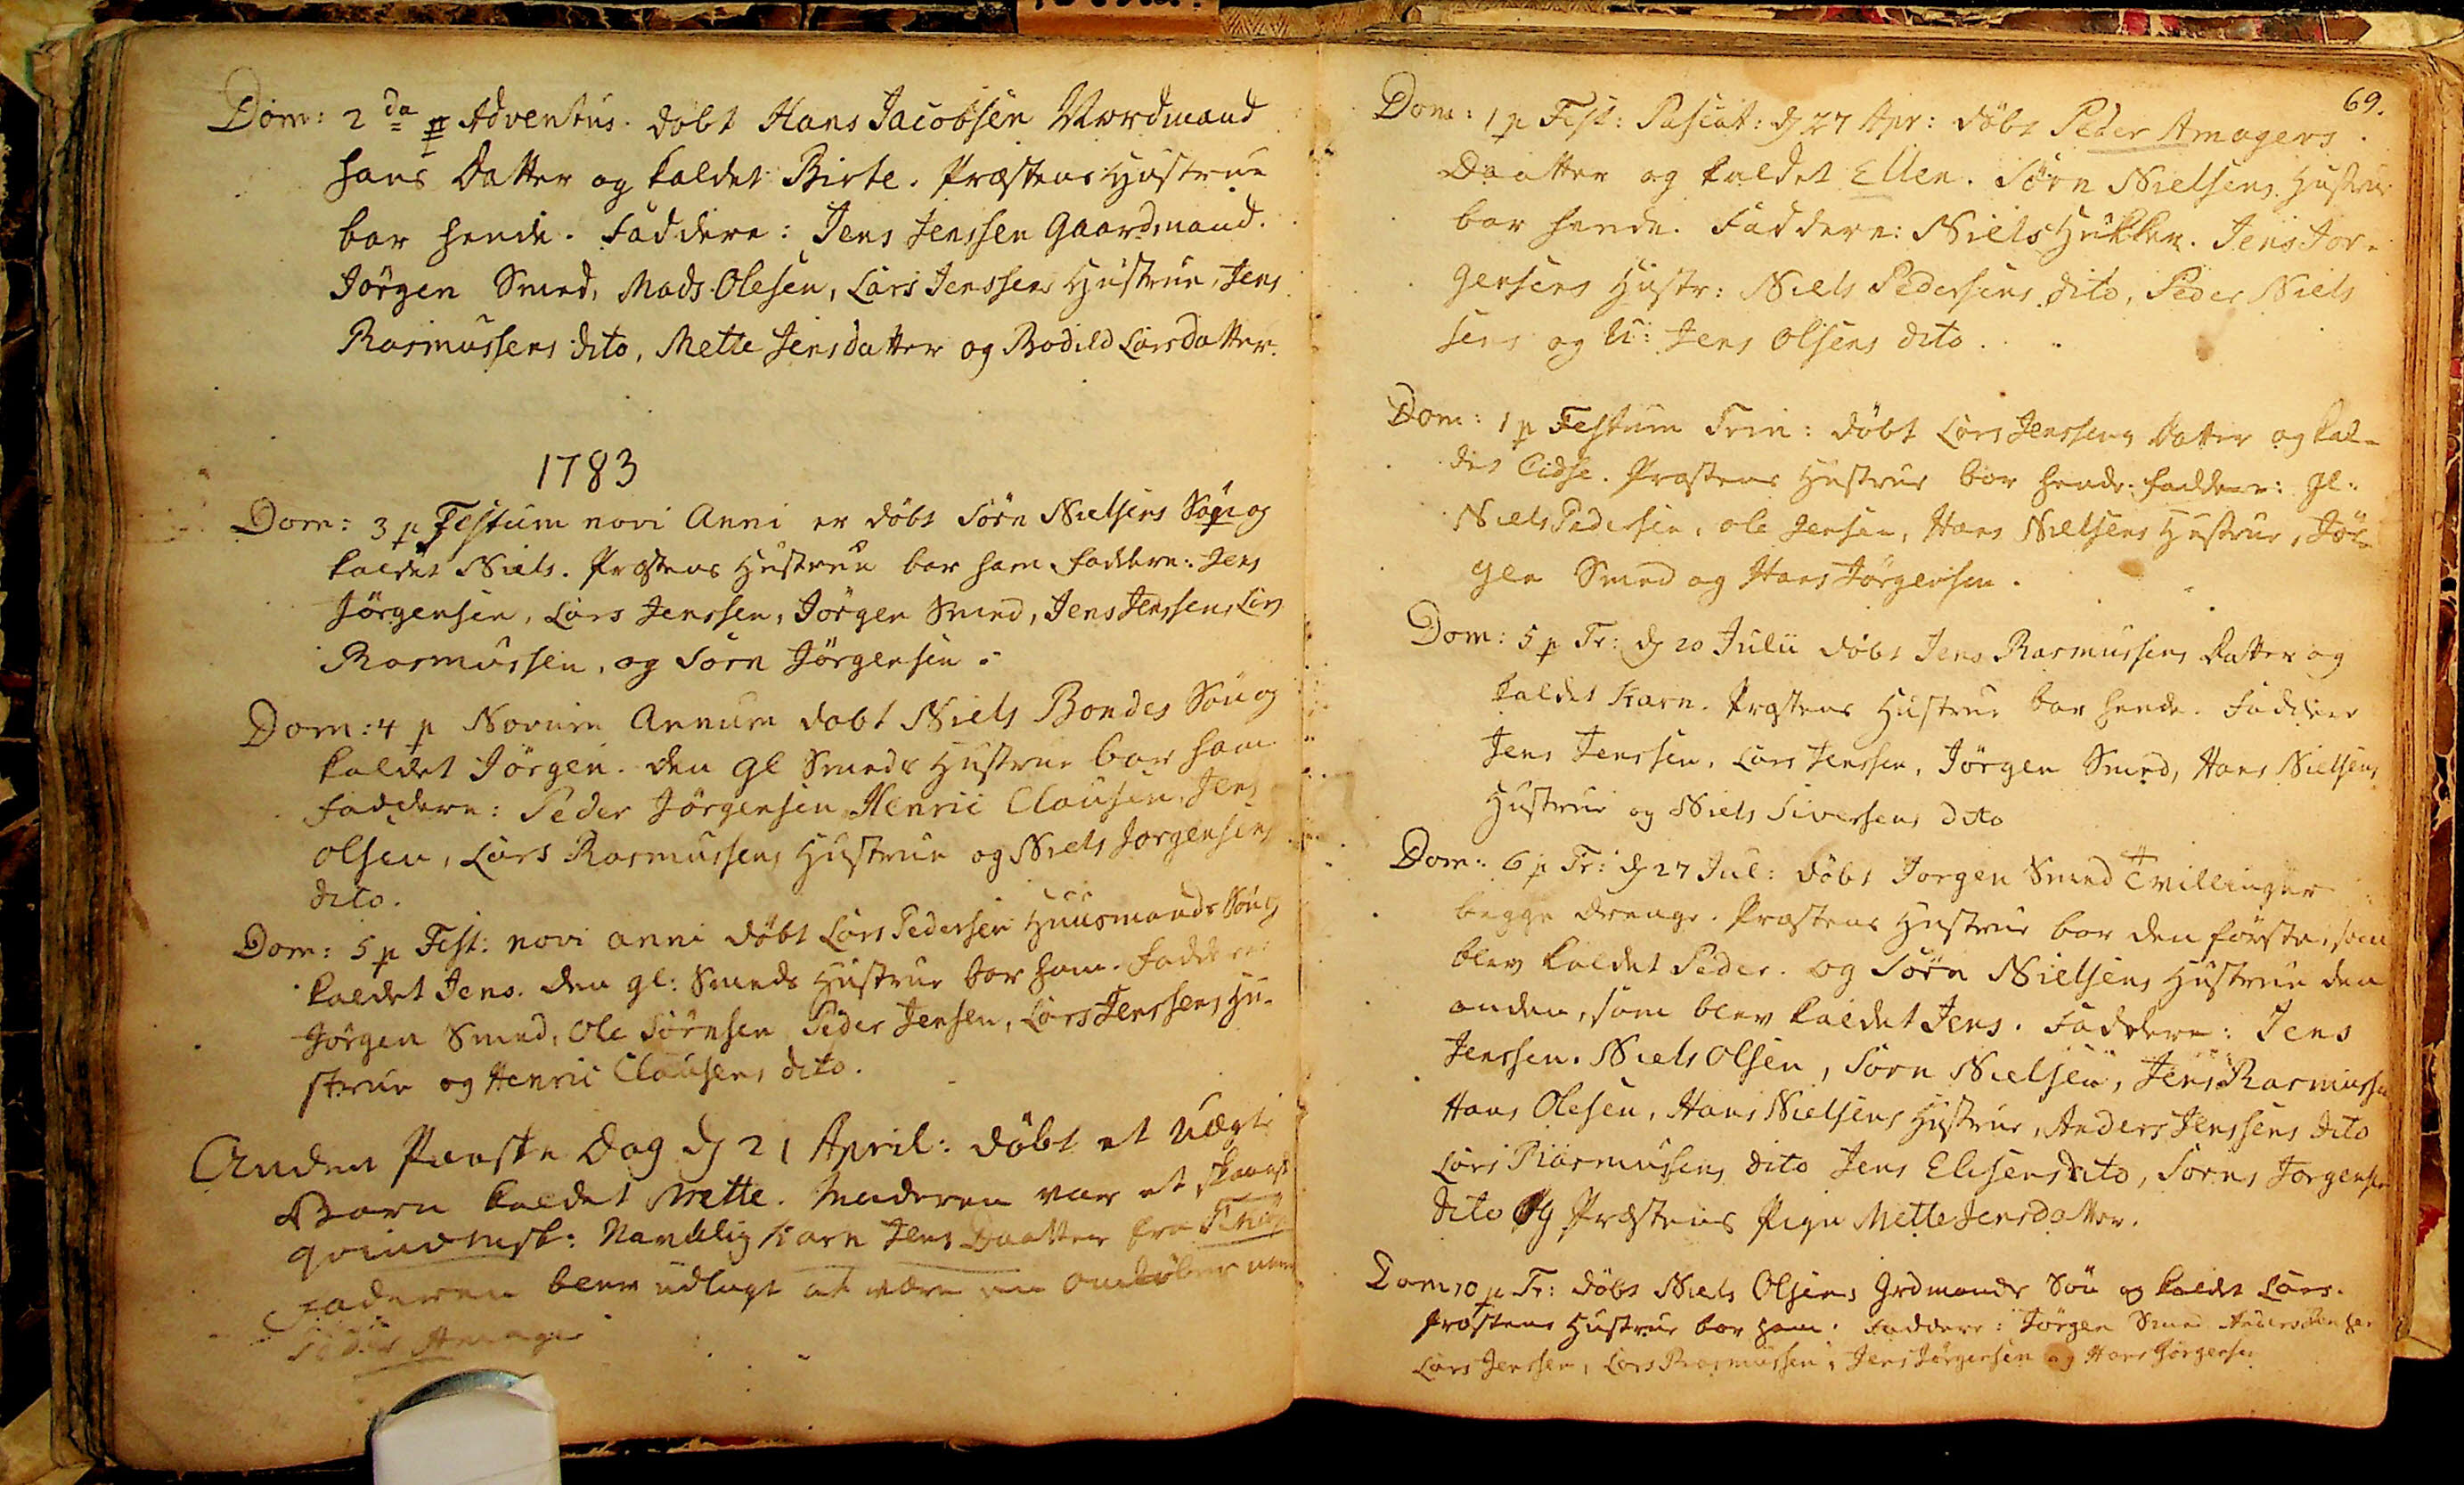Dom: 2da p Adventus døbt Hans Jacobsen Nordmand
hans Datter og kaldet Birte. Præstens Hustrue
bar hende. Faddere: Jens Jenssen Gaardmand.
Jørgen Smed, Mads Olesen, Lars Jenssens Hustrue, Jens
Rasmussens dito, Mette Jensdatter og Bodild Larsdatter.
1783
Dom: 3 p Festum novi Anni er døbt Sørn Nielsens Søn og
kaldet Niels. Præstens Hustrue bar ham. Faddere: Jens
Jørgensen, Lars Jenssen, Jørgen Smed, Jens Jenssen, Lars
Rasmussen, og Sørn Jørgensen:
Dom: 4 p Novum Annum døbt Niels Bondes Søn og 
kaldet Jørgen. Den gl Smeds Hustrue bar ham.
Faddere: Peder Jørgensen, Henric Clausen, Jens
Olsen, Lars Rasmussens Hustrue og Niels Jørgensens
dito.
Dom: 5 p Fest: novi Anni døbt Lars Pedersen Huusmands Søn og
kaldet Jens. Den gl: Smeds Hustrue bar ham. Faddere:
Jørgen Smed, Ole Sørnsen, Peder Jensen, Lars Jenssens Hu¬
strue og Henric Clausens dito.
Anden Paaske Dag d 21 April: døbt et uægte
Barn kaldet Mette. Moderen var et skaansk
qvindmsk: Navnlig Karen Jens Daatter fra Kiøge##
Faderen blev udlagt at være en omløber nem##
Peder Amager
69.
Dom: 1 p Fest: Pascat: d 27 Apr: døbt Peder Amagers
Daatter og kaldet Ellen. Sørn Nielsens Hustrue
bar hende. Faddere: Niels Hukker. Jens Jør¬
gensens Hustr: Niels Pedersens dito, Peder Niels
sens og U: Jens Olsens dito.
Dom: 1 p Festum Trin: døbt Lars Jenssens Datter og kal¬
det Cidse. Præstens Hustrue bar hende. faddere: gl:
Niels Pedersen, Ole Jensen, Hans Nielsens Hustrue, Jør¬
gen Smed og Hans Jørgensen.
Dom: 5 p Tr: d 20 Julii døbt Jens Rasmussens Datter og
kaldet Karn. Præstens Hustrue bar hende. Faddere
Jens Jenssen, Lars Jenssen, Jørgen Smed, Hans Nielsens
Hustrue og Niels Siversens dito
Dom: 6 p Tr: d 27 Jul: døbt Jørgen Smed Tvillinger
begge Drenge. Præstens Hustrue bar den første, som
blev kaldet Peder. og Sørn Nielsens Hustrue den
anden, som blev kaldet Jens. Faddere: Jens 
Jenssen. Niels Olsen, Sørn Nielsen, Jens Rasmussen
Hans Olesen, Hans Nielsens Hustrue, Anders Jenssens dito
Lars Rasmussens dito Jens Elisens dito, Sørns Jørgensens
dito og Præstens Pige Mette Jensdatter.
Dom 10 p Tr: døbt Niels Olsens Grdmands Søn og kaldet Lars.
Præstens Hustrue bar ham. Faddere: Jørgen Smed. Anders Jensen
Lars Jenssen, Lars Rasmussen, Jens Jørgensen og Hans Jørgensen
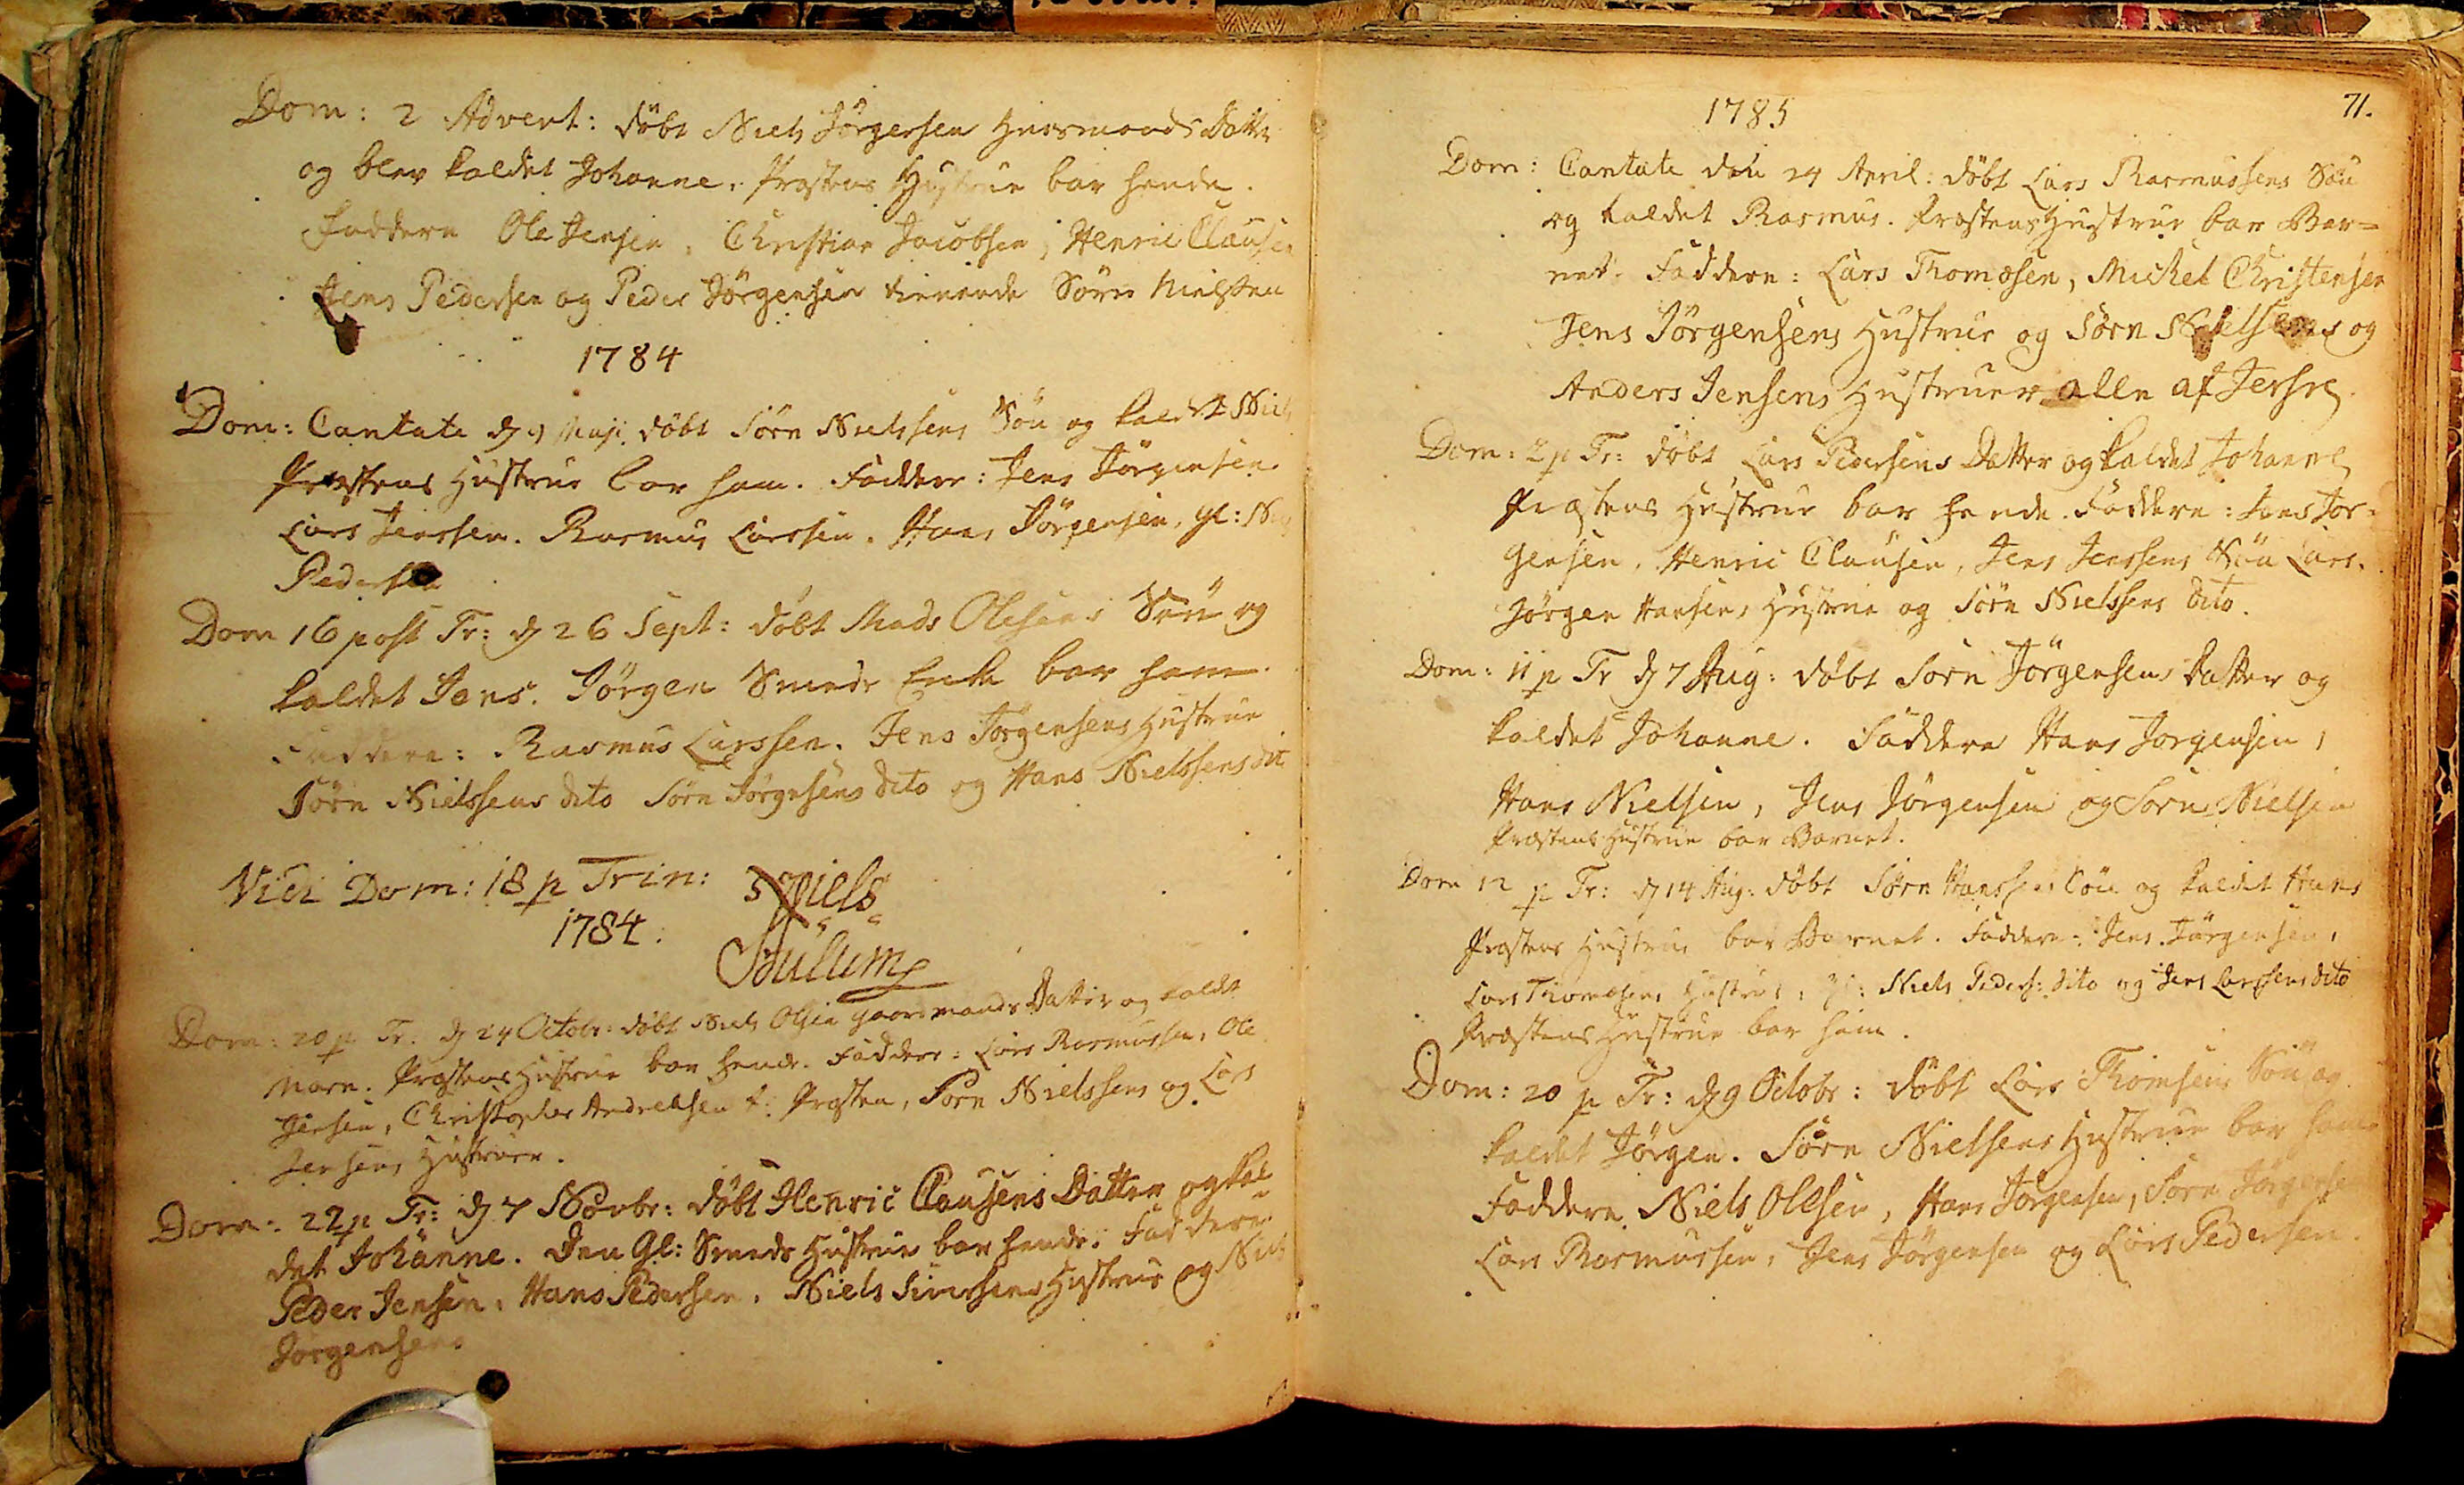Dom: 2 Advent: døbt Niels Jørgensen Huusmands Datter
og blev kaldet Johanne. Præstens Hustrue bar hende.
Faddere Ole Jensen, Christian Jacobsen, Henric Clausen
Jens Pedersen og Peder Jørgensen tienende Sørn Nielsen
1784
Dom: Cantate d 9 Maji døbt Sørn Nielssens Søn og kaldet Niels
Præstens Hustrue bar ham. Faddere: Jens Jørgensen
Lars Jenssen. Rasmus Larssen. Hans Jørgensen, gl: Niels
Pedersen
Dom: 16 post Tr: d 26 Sept: døbt Mads Olesens Søn og
kaldet Jens. Jørgen Smeds Enke bar ham.
Faddere: Rasmus Larssen. Jens Jørgensens Hustrue
Sørn Nielssens dito Sørn Jørgnsens dito og Hans Nielssens dito
Vidi Dom: 18 p Trin:
1784
Niels
Aulum
Dom: 20 p Tr: d 24 Octobr: døbt Niels Olsen Gaardmands Datter og kaldet
Marn. Præstens Hustrue bar hende. Faddere: Lars Rasmussen, Ole
Jensen, Christopher Andreasen t: Præsten, Sørn Nielssens og Lars
Jensens Hustruer.
Dom: 22 p Tr: d 7 Novbr: døbt Henric Clausens Datter og kal¬
det Johanne. Den Gl: Smeds Hustrue bar hende. Faddere:
Peder Jensen, Hans Pedersen, Niels Siversens Hustrue og Niels
Jørgensens
71.
1785
Dom: Cantate den 24 April: døbt Lars Rasmussens Søn
og kaldet Rasmus. Præstens Hustrue bar Bar¬
net. Faddere: Lars Thomæsen, Michel Christensen
Jens Jørgensens Hustrue og Sørn Nielsens og
Anders Jensens Hustruer alle af Jersie.
Dom: 2 p Tr: døbt Lars Pedersens Datter og kaldet Johanne
Præstens Hustrue bar hende. Faddere: Jens Jør¬
gensen, Henric Clausen, Jens Jenssens Søn Lars,
Jørgen Hansens Hustrue og Sørn Nielssens dito.
Dom: 11 p Tr d 7 Aug: døbt Sørn Jørgensens Datter og
kaldet Johanne. Faddere Hans Jørgensen,
Hans Nielsen, Jens Jørgensen og Sørn Nielsen
Præstens Hustrue bar Barnet.
Dom 12 p Tr: d 14 Aug: døbt Sørn Hanssens Søn og kaldet Hans
Præstens Hustrue bar Barnet. Faddere: Jens Jørgensen,
Lars Thomæsens Hustrue, Gl: Niels Peders: dito og Jens Larssens dito
Præstens Hustrue bar ham.
Dom: 20 p Tr: d 9 Octobr: døbt Lars Thomsens Søn og
kaldet Jørgen. Sørn Nielsens Hustrue bar ham.
Faddere. Niels Olesen, Hans Jørgensen, Sørn Jørgensen
Lars Rasmussen, Jens Jørgensen og Lars Pedersen.